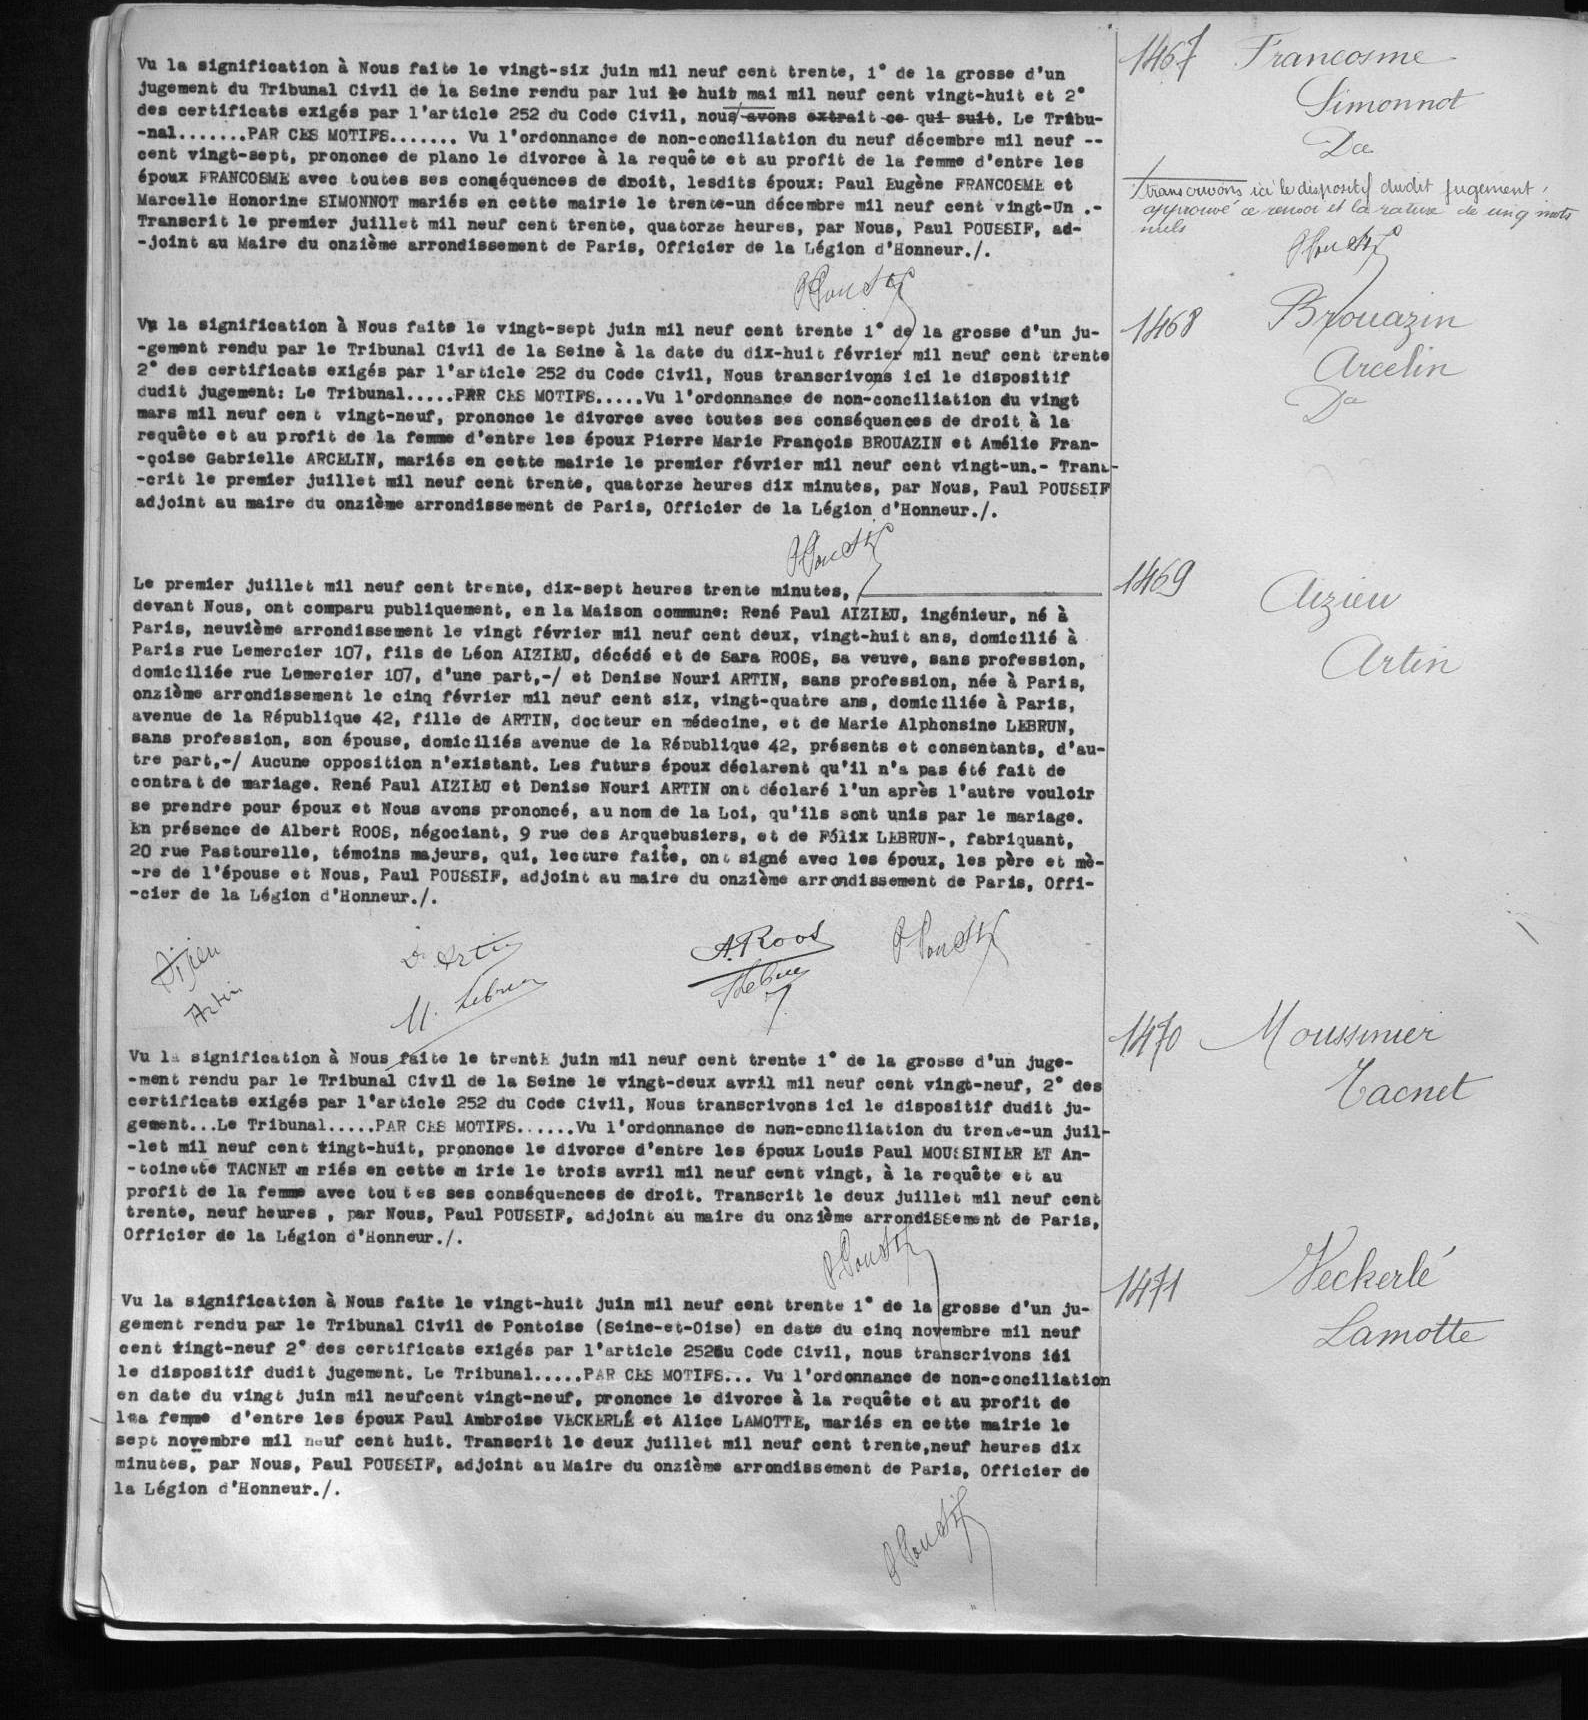Vu la signification à Nous faite le vingt-six juin mil neuf cent trente, 1° de la grosse d'un
jugement du Tribunal Civil de la Seine rendu par lui le huit mai mil neuf cent vingt-huit et 2°
des certificats exigés par l'article 252 du Code Civil, nous avons extrait ce qui suit. Le Tribu-
-nal..... PAR CES MOTIFS..... Vu l'ordonnance de non-conciliation du neuf décembre mil neuf -
cent vingt-sept, prononce de plano le divorce à la requête et au profit de la femme d'entre les
époux FRANCOSME avec toutes ses conséquences de droit, lesdits époux: Paule Eugène FRANCOSME et
Marcelle Honorine SIMONNOT mariés en cette mairie le trente-un décembre mil neuf cent vingt-Un .-
Transcrit le premier juillet mil neuf cent trente, quatorze heures, par Nous, Paul POUSSIF, ad-
-joint au Maire du onzième arrondissement de Paris, Officier de la Légion d'Honneur ./.
Vu la signification à Nous faite le vingt-sept juin mil neuf cent trente 1° de la grosse d'un ju-
-gement rendu par le Tribunal Civil de la Seine à la date du dix-huit février mil neuf cent trente
2° des certificats exigés par l'article 252 du Code Civil, Nous transcrivons ici le dispositif
dudit jugement: Le Tribunal.... PAR CES MOTIFS.... Vu l'ordonnance de non-conciliation du vingt
mars mil neuf cen t vingt-neuf, prononce le divorce avec toutes ses conséquences de droit à la
requête et au profit de la femme d'entre les époux Pierre Maire François BROUAZIN et Amélie Fran-
-çoise Gabrielle ARCELIN, mariés en cette mairie le premier février mil neuf cent vingt-un .-Trans-
-crit le premier juillet mil neuf cent trente, quatorze heures dix minutes, par Nous, Paul POUSSIF
adjoint au maire du onzième arrondissement de Paris, Officier de la Légion d'Honneur ./.
Le premier juillet mil neuf cent trente, dix-sept heures trente minutes, -
devant Nous, ont comparu publiquement, en la Maison commune: René Paul AZIEU, ingénieur, né à
Paris, neuvième arrondissement le vingt février mil neuf cent deux, vingt-huit ans, domicilié à
Paris rue Lemercier 107, fils de Léon AIZIEU, décédé et de Sara ROOS, sa veuve, sans profession,
domiciliée rue Lemercier 10, d'une part ,-/ et Denise Nouri ARTIN, sans profession, née à Paris,
onzième arrondissement le cinq février mil neuf cent six, vingt-quatre ans, domiciliée à Paris,
avenue de la République 42, fille de ARTIN, docteur en médecine, et de Marie Alphonsine LEBRUN,
sans profession, son épouse, domiciliés avenue de la République 42, présents et consentants, d'au-
tre part .-/ Aucune opposition n'existant. Les futurs époux déclarent qu'il n'a pas été fait de
contrat de mariage. René Paul AIZIEU et Denise Nouri ARTIN ont déclaré l'un après l'autre vouloir
se prendre pour époux et Nous avons prononcé, au nom d ela Loi, qu'ils sont unis par le mariage.
En présence de Albert ROOS, négociant, 9 rue des Arquebusiers, et de Félix LEBUN -, fabriquant,
20 rue Pastourelle, témoins majeurs, qui, lecture faite, ont signé avec les époux, les père et mè-
-re de l'épouse et Nous, Paul POUSSIF, adjoint au maire du onzième arrondissement de Paris, Offi-
-cier de la Légion d'Honneur ./.
Vu la signification à Nous faite le trente juin mil neuf cent trente 1° de la grosse d'un juge-
-ment rendu par le Tribunal Civil de la Seine le vingt-deux avril mil neuf cent vingt-neuf, 2° des
certificats exigés par l'article 252 du Code Civil, Nous transcrivons ici le dispositif dudit ju-
gement .... Le Tribunal ... PAR CES MOTIFS ... Vu l'ordonnance de non-conciliation du tren e-un juil-
-let mil neuf cent * ingt-huit, prononce le divorce d'entre les époux Louis Paul MOUSSINIER et An-
-toinette TACNET m riés en cette m irie le trois avril mil neuf cent vingt, à la requête et au
profit de la femme avec toutes ses conséquences de droit. Transcrit le deux juillet mil neuf cent
trente, neuf heures, par Nous, Paul POUSSIF, adjoint au maire du onzième arrondiSSement de Paris,
Officier de la Légion d'Honneur ./.
Vu la signification à Nous faite le vingt-huit juin mil neuf cent trente 1° de la frosse d'un ju-
gement rendu par le Tribunal Civil de Pontoise (Seine-et-Oise) en date du cinq novembre mil neuf
cent *ingt-neuf 2° des certificats exigés par l'article 252du Code Civil, nous transcrivons ici
le dispositif dudit jugement. Le Tribunal..... PAR CES MOTIFS... Vu l'ordonnance de non-conciliation
en date du vingt juin mil neuf cent vingt-neuf, prononce le divorce à la requête et au profit de
l*a femme d'entre les époux Paul Ambroise VECKERLE et Alice LAMOTTE, mariés en cette mairie le
sept novembre mil neuf cent huit. Transcrit le deux juillet mil neuf cent trente, neuf heures dix
minutes, par Nous, Paul POUSSIF, adjoint au Maire du onzième arrondissement de Paris, Officier de
la Légion d'Honneur ./.
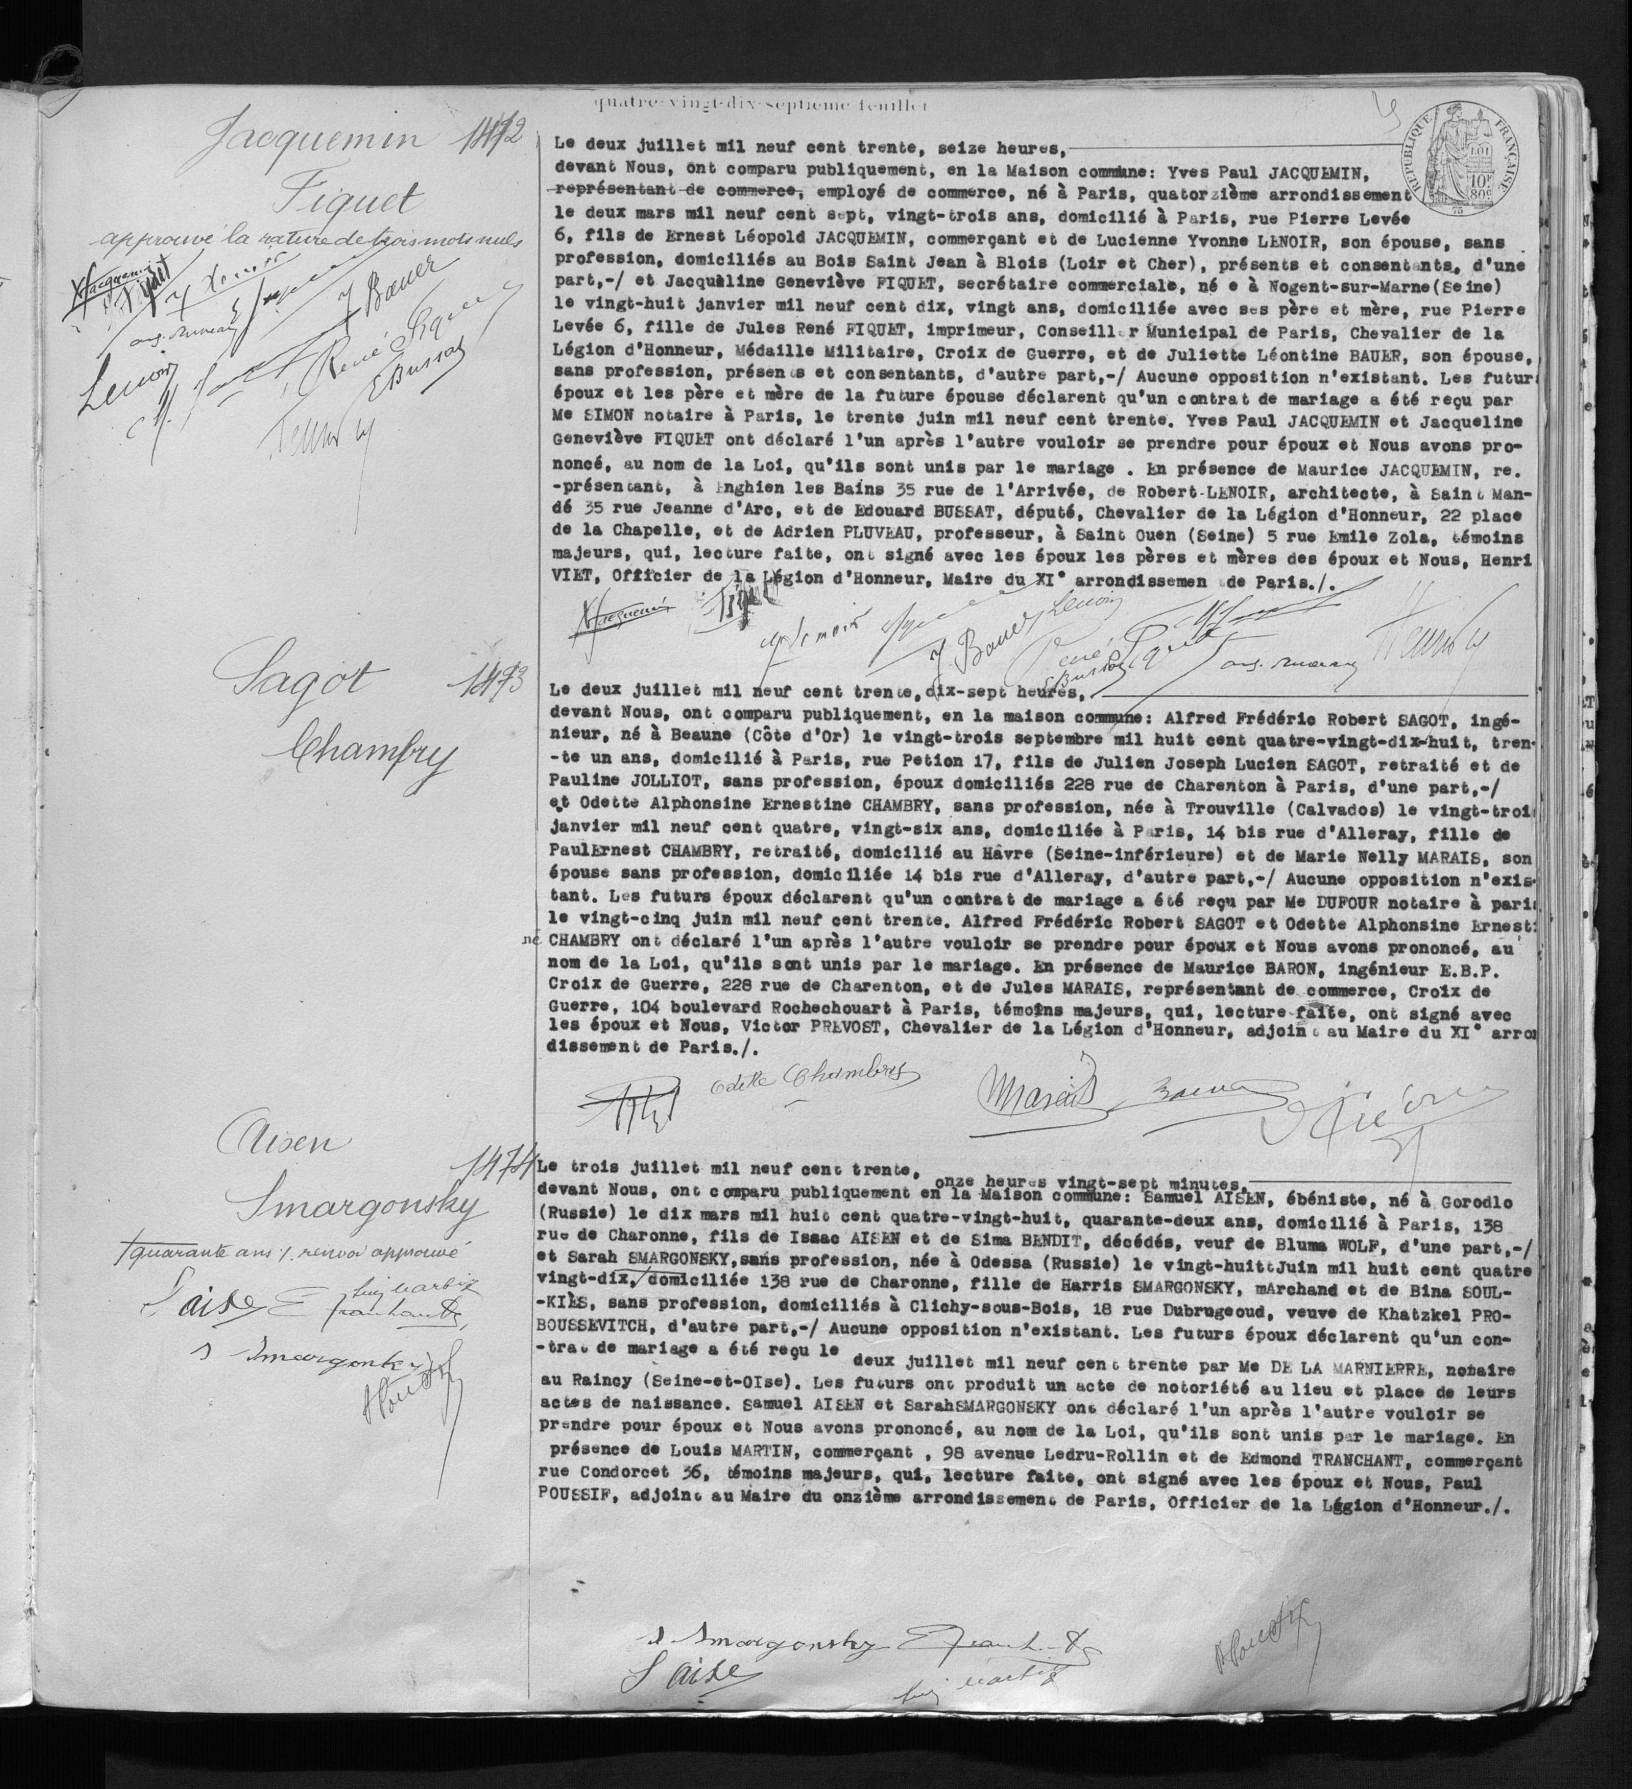Le deux juillet mil neuf cent trente, seize heures, -
devant Nous, ont comparu publiquement, en la Maison commune: Yves Paul JACQUEMIN,
représentant de commerce, employé de commerce, né à Paris, quatorzième arrondissement
le deux mars mil neuf cent sept, vingt-trois ans, domicilié à Paris, rue Pierre Levée
6, fils de Ernest Léopold JACQUEMIN, commerçant et de Lucienne Yvonne LENOIR, son épouse, sans
profession, domiciliés au Bois Saint Jean à Blois (Loir et Cher), présents et consentants, d'une
part ,-/ et Jacqueline Geneviève FIQUET, secrétaire commerciale, né o à Nogent-sur-Marne (Seine)
le vingt-huit janvier mil neuf cent dix, vingt ans, domiciliée avec ses père et mère, rue Pierre
Levée 6, fille de Jules René FIQUET, imprimeur, Conseiller Municipal de Paris, Chevalier de la
Légion d'Honneur, Médaille Militaire, Croix de Guerre, et de Juliette Léontine BAUER, son épouse,
sans profession. présents et consentants, d'autre part, -/ Aucune opposition n'existant. Les futur
époux et les père et mère de la future épouse déclarent qu'un contrat de mariage a été reçu par
Me SIMON notaire à Paris, le trente juin mil neuf cent trente. Yves Paul JACQUEMIN et Jacqueline
Geneviève FIQUET ont déclaré l'un après l'autre vouloir se prendre pour époux et Nous avons pro-
noncé, au nomde la Loi, qu'ils sont unis par le mariage. En présence de Maurice JACQUEMIN, re.
-présentant, à Enghien les Bains 35 rue de l'Arrivée, de Robert LENOIR, architecte, à Saint Man-
dé 35 rue Jeanne d'Arc. et de Edouard BUSSAT, député, Chevalier de la Légion d'Honneur, 22 place
de la Chapelle, et de Adrien PLUVEAU, professeur, à Saint Ouen (Seine) 5 rue Emile Zola, témoins
majeurs, qui, lecture faite, ont signé avec les époux les pères et mères des époux et Nous, Henri
VIET, Officier de la Légion d'Honneur, Maire du XI ° arrondissemen de Paris ./.
Le deux juillet mil neuf cent trente, dix-sept heures, -
devant Nous, ont comparu publiquement, en la maison commune: Alfred Frédéric Robert SAGOT, ingé-
nieur, né à Beaune (Côte d'Or) le vingt-trois septembre mil huit cent quatre-vingt-dix-huit, tren-
-te un ans, domicilié à Paris, rue Petion 17, fils de Julien Joseph Lucien SAGOT, retraité et de
Pauline JOLLIOT, sans profession, époux domiciliés 228 rue de Charenton à Paris, d'une part .-/
et Odette Alphonsine Ernestine CHAMBRY, sans profession, née à Trouville (Calvados) le vingt-troi
janvier mil neuf cent quatre, vingt-six ans, domiciliée à Paris, 14 bis rue d'Alleray, fille de
Paul Ernest CHAMBRY, retraité, domicilié au Havre (Seine-Inférieure) et de Marie Nelly MARAIS, son
épouse sans profession, domicilié 14 bis rue d'Alleray, d'autre part ,-/ Aucune opposition n'exis-
tant. Les futurs époux déclarent qu'un contrat de mariage a été reçu par Me DUFOUR notaire à pari
le vingt-cinq juin mil neuf cent trente. Alfred Frédéric Robert SAGOT et Odette Alphonsine Ernest
CHAMBRY ont déclaré l'un après l'autre vouloir se prendre pour époux et Nous avons prononcé, au
nom de la Loi, qu'ils sont unis par le mariage. En présence de Maurice BARON, ingénieur E. B. P.
Croix de Guerre, 228 rue de Charenton, et de Jules MARAIS représentant de commerce, Croix de
Guerre, 104 boulevard Rochechouart à Paris, témoins majeurs, qui, lecture faite, ont signé avec
les époux et Nous, Victor PREVOST, Chevalier de la Légion d'Honneur, adjoin au Maire du XI ° arro
dissement de Paris ./.
Le trois juillet mil neuf cent trente, onze heures vingt-sept minutes ,-
devant Nous, ont comparu publiquement en la Maison commune: Samuel AISEN, ébéniste, né à Gorodlo
(Russie) le dix mars mil huit cent quatre-vingt-huit, quarante-deux ans, domicilié à Paris, 138
rue de Charonne, fils de Isaac AISEN et de Sima BENDIT, décédés, veuf de Bluma WOLF, d'une part ,-/
et Sarah SMARGONSKY, sans profession, née à Odessa (Russie) le vingt-huit Juin mil huit cent quatre
vingt-dix, domiciliée 138 rue de Charonne, fille de Harris SMARGONSKY, mArchand et de Bina SOUL-
-KIES, sans profession, domiciliés à Clichy-sous-Bois, 18 rue Dubrugeoud, veuve de Khatzkel PRO-
BOUSSEVITCH, d'autre part ,-/ Aucune opposition n'existant. Les futurs époux déclarent qu'un con-
-tra de mariage a été reçu le deux juillet mil neuf cent trente par Me DE LA MARNIERRE, notaire
au Raincy (Seine-et-OIse). Les futurs ont produit un acte de notoriété au lieu et place de leurs
actes de naissance. Samuel AISEN et Sarah SMARGONSY ont déclaré l'un après l'autre vouloir se
prendre pour époux et Nous avons prononcé, au nom de la Loi, qu'ils sont unis par le mariage. En
présence de Louis MARTIN, commerçant, 98 avenue Ledru-Rollin et de Edmon TRANCHANT, commerçant
rue Condorcet 36, témoins majeurs, qui, lecture faite, ont signé avec les époux et Nous, Paul
POUSSIF, adjoint au Maire du onzième arrondissement de Paris, Officier de la Lggion d'Honneur ./.